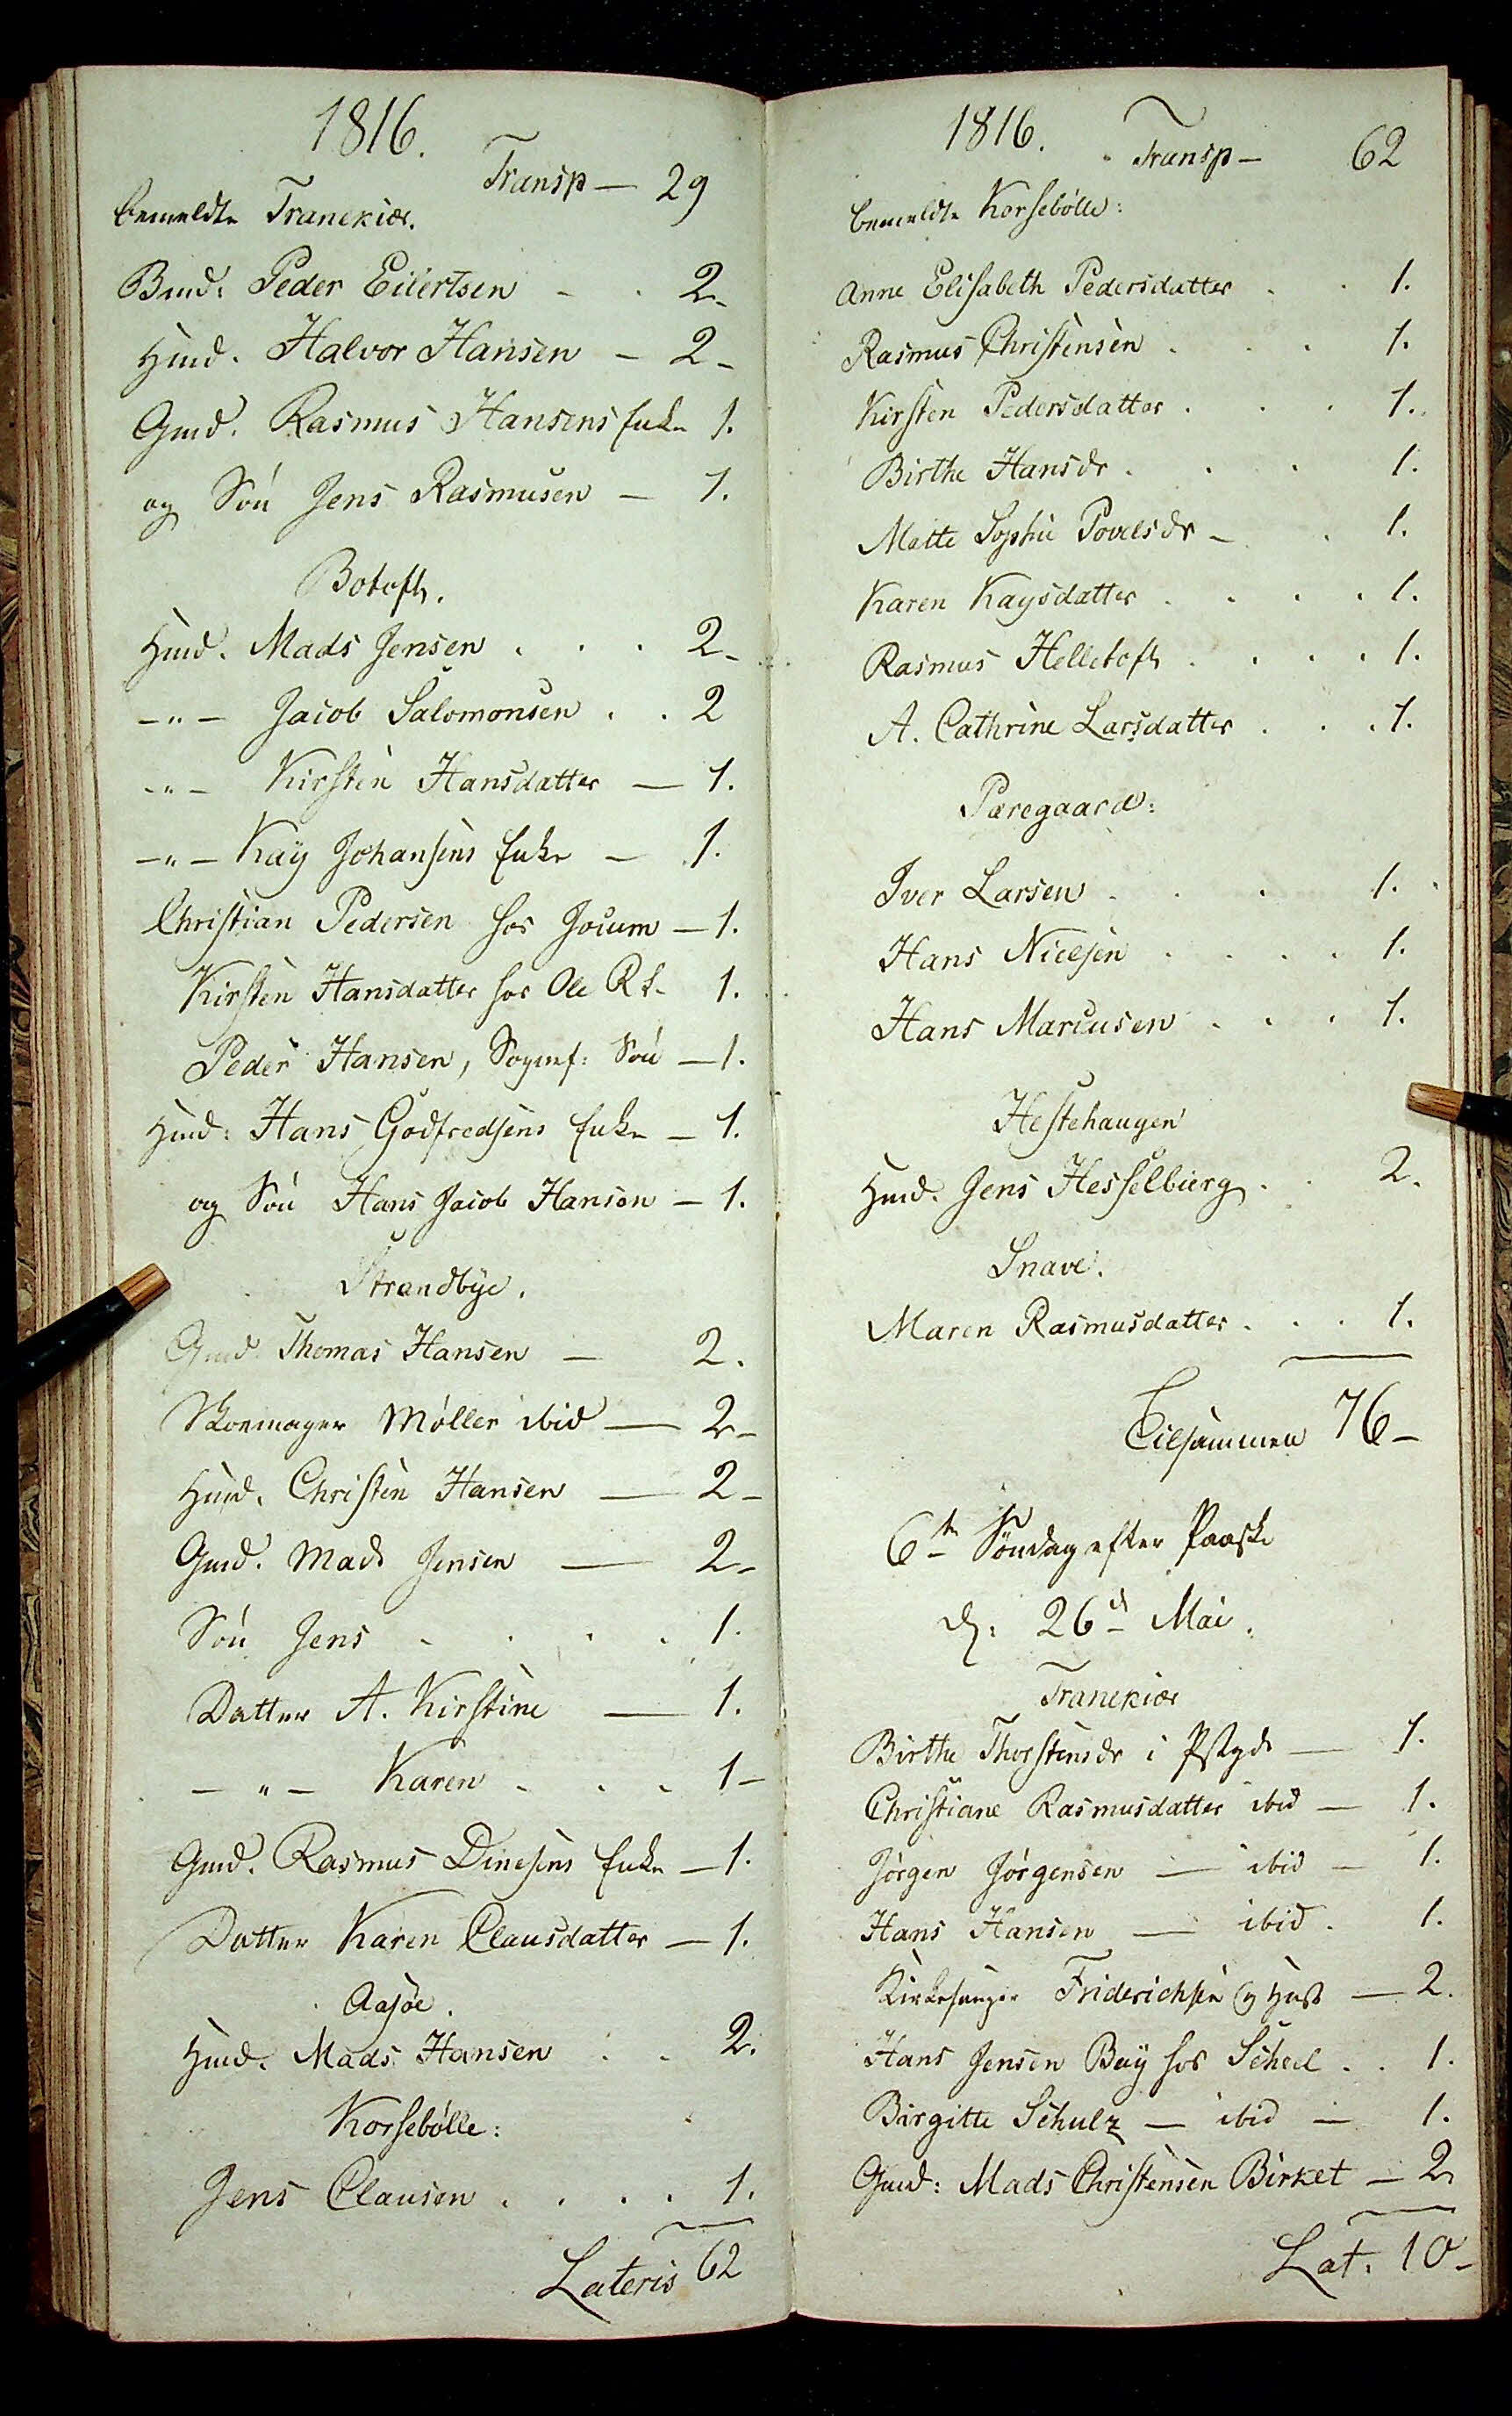1816
Transp - 29
bemeldte Tranekiær.
Bmd: Peder Eilertsen - . 2
Hmd: Halvor Hansen - 2
Gmd. Rasmus Hansens Enke 1.
og Søn Jens Rasmusen - 1.
Botoft.
Hmd. Mads Jensen . . . 2.
-"- Jacob Salomonsen . . 2
-"- Kirsten Hansdatter - 1.
-"- Kay Johansens Enke - 1.
Christian Pedersen hos Jocum -1.
Kirsten Hansdatter hos Ole R## 1.
Peder Hansen, Sognef: Søn - 1.
Hmd: Hans Godfredsens Enke - 1.
og Søn Hans Jacob Hansen - 1.
Strandbye.
Gmd. Thomas Hansen - 2.
Skoemager Møller ibid - 2-
Hmd. Christen Hansen - 2.
Gmd. Mads Jensen - 2
Søn Jens . . . . 1.
Datter A. Kirstine - 1.
-"- Karen . . . 1-
Gmd. Rasmus Dinesens Enke - 1.
Datter Karen Clausdatter - 1.
Aasøe
Hmd. Mads Hansen . . 2.
Korsebølle.
Jens Clausen . . . . 1.
Lateris 62
1816.
Transp - 62
bemeldte Korsebølle:
Anne Elisabeth Pedersdatter . . 1.
Rasmus Christensen . . . 1.
Kirsten Pedersdatter . . . 1.
Birthe Hansdr . . . 1.
Mette Sophie Povelsdr - . 1.
Karen Kaysdatter . . . . 1.
Rasmus Helletoft . . . . 1.
A. Cathrine Larsdatter . . . 1.
Pæregaard:
Iver Larsen . . . 1.
Hans Nielsen . . . . 1.
Hans Marcusen . . . 1.
Hestehaugen
Hmd: Jens Hesselbierg . . 2.
Snave.
Maren Rasmusdatter . . . 1.
Tilsammen 76-
6te Søndag efter Paaske
d: 26de Mai.
Tranekiær
Birthe Thorstensdr i Pstgd - 1.
Christiane Rasmusdatter ibid - 1.
Jørgen Jørgensen - ibid - 1.
Hans Hansen - ibid. 1.
Kirkesanger Friderichsen og Hust - 2.
Hans Jensen Bay hos Scheel . .1.
Birgitte Schulz - ibid - 1.
Gund: Mads Christensen Birket - 2
Lat. 10-
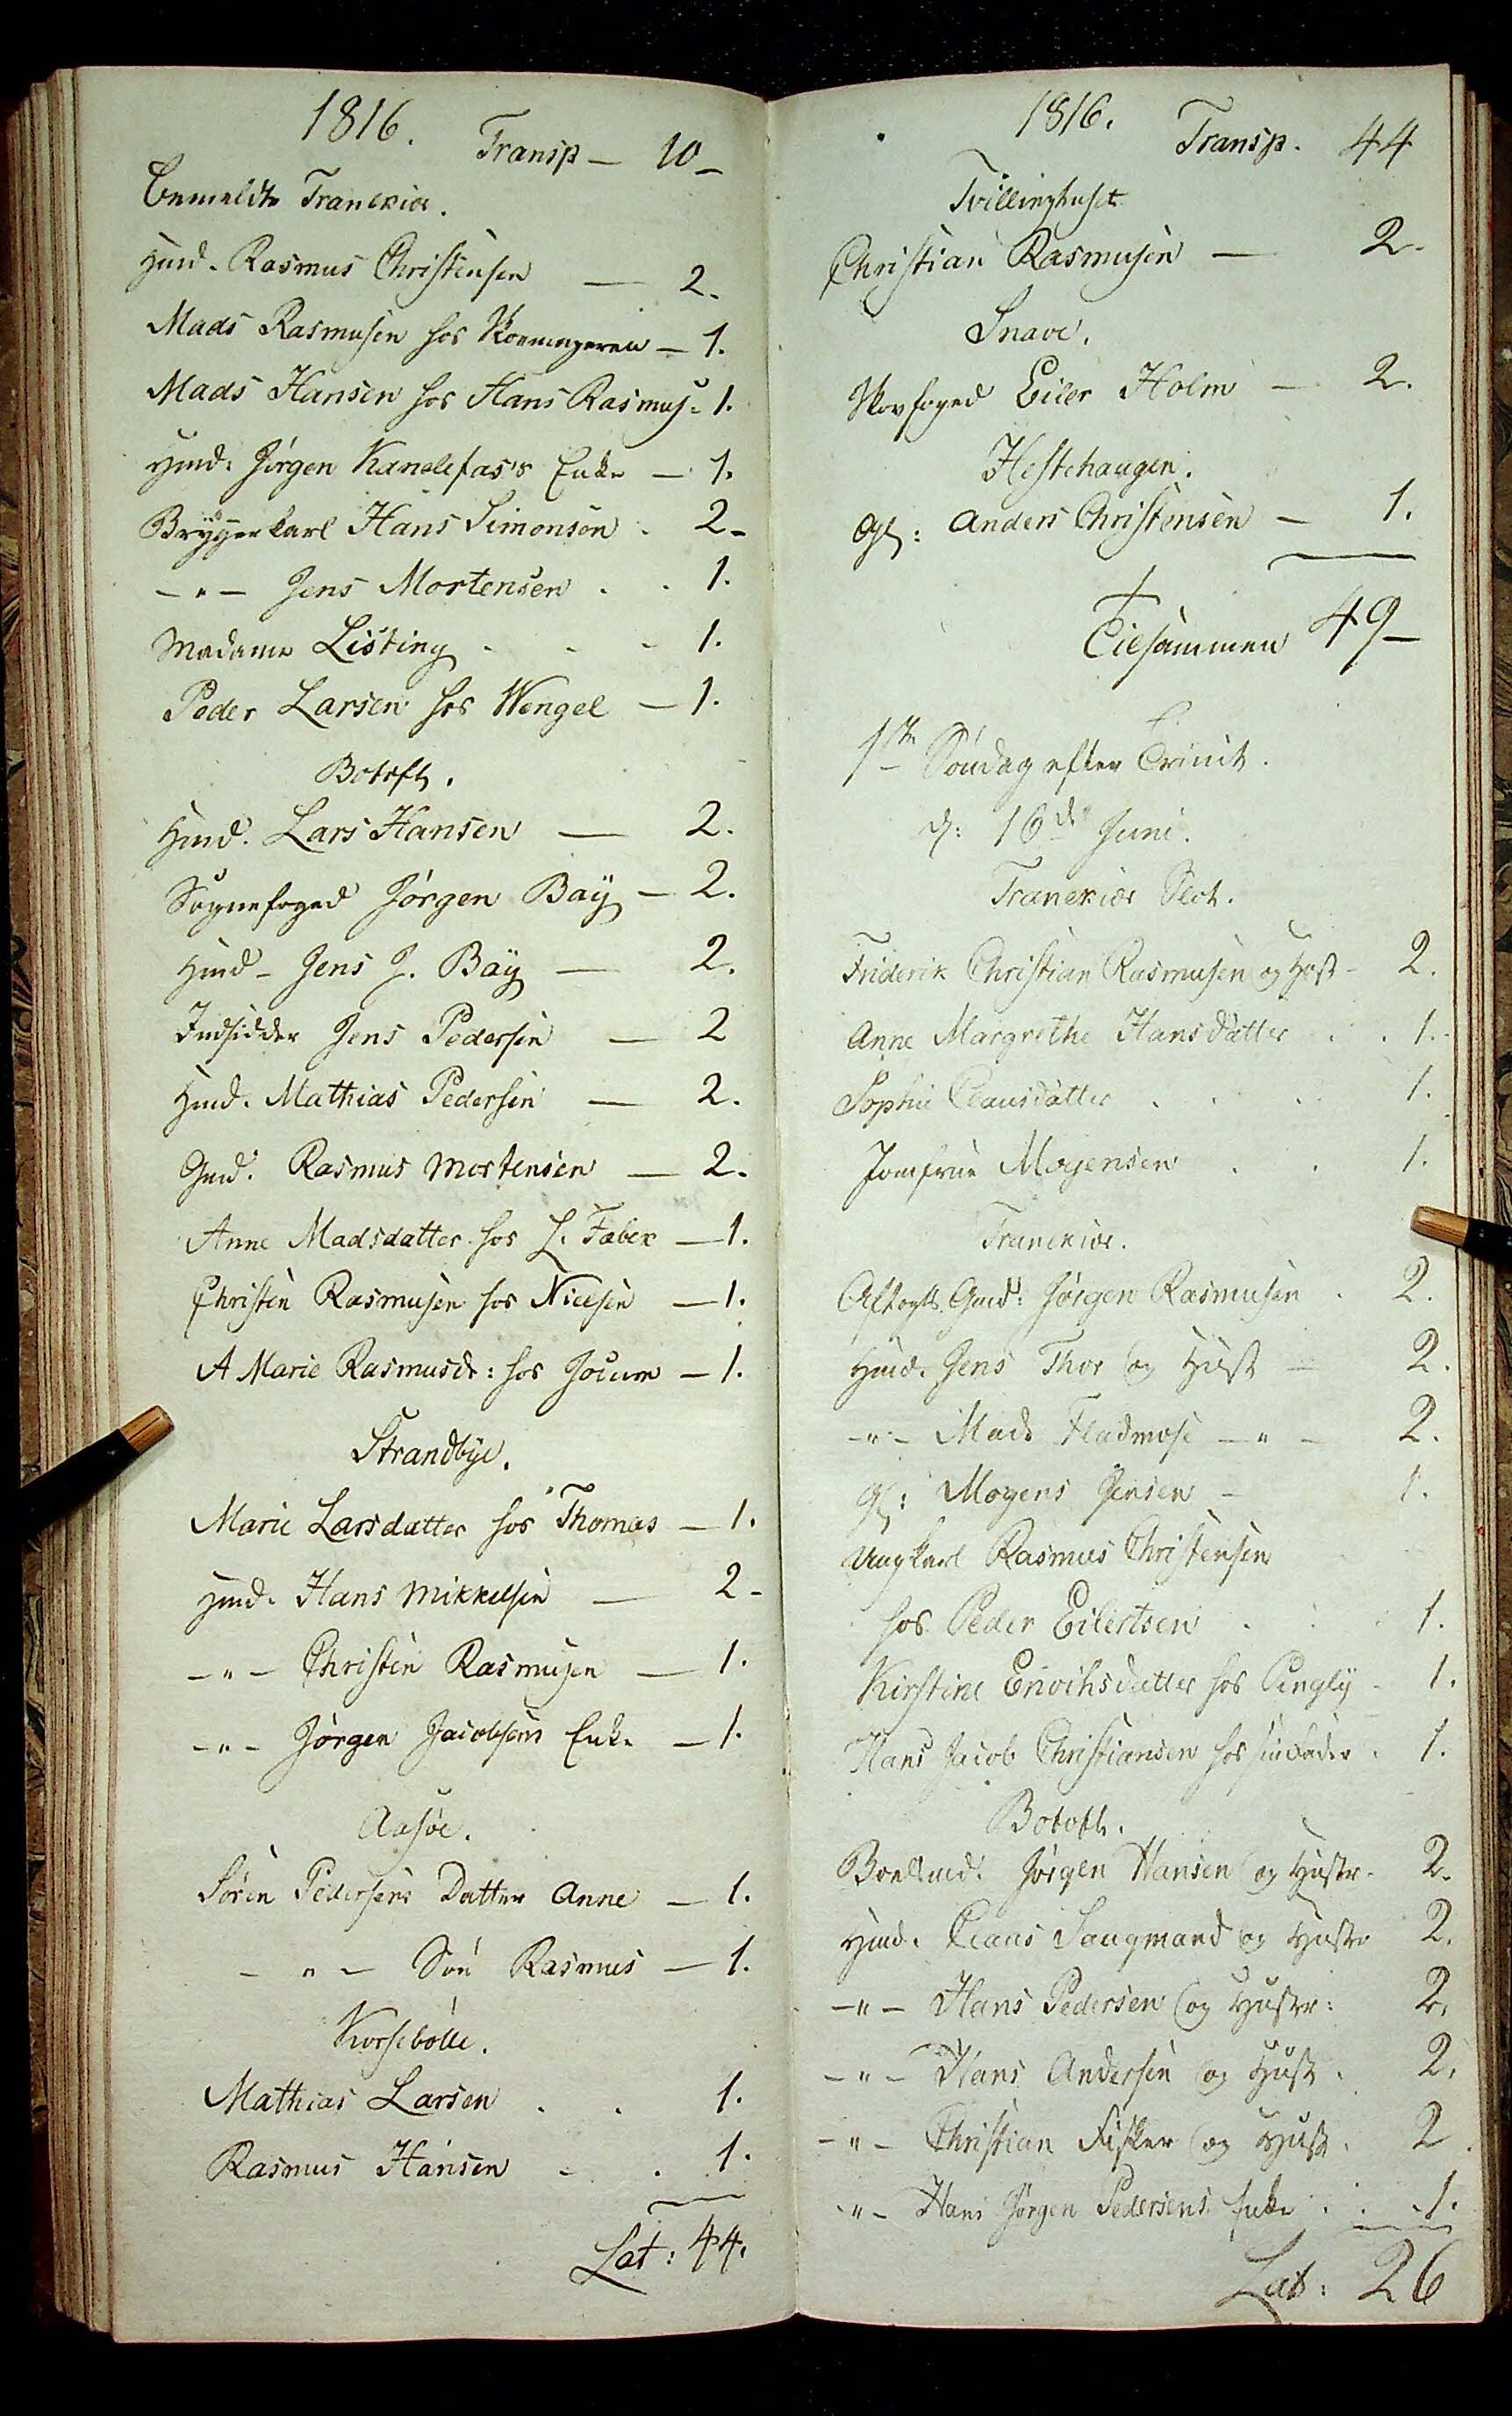1816.
Transp - 10-
bemeldte Tranekiær.
Hmd. Rasmus Christensen - 2
Mads Rasmusen hos Skoemageren - 1.
Mads Hansen hos Hans Rasmus . 1.
Hmd: Jørgen Kanelefas's Enke - 1.
Bryggerkarl Hans Simonsen . 2-
-"- Jens Mortensen . . 1.
Madame Listing . . 1.
Peder Larsen hos Wengel - 1.
Botoft.
Hmd. Lars Hansen - 2.
Sognefoged Jørgen Bay - 2.
Hmd- Jens J. Bay - 2.
Indsidder Jens Pedersen 2
Hmd. Mathias Pedersen - 2.
Grd. Rasmus Mortensen - 2.
Anne Madsdatter hos L. Fæber - 1.
Christen Rasmusen hos Nielsen - 1
A. Marie Rasmusdr: hos Jocum - 1.
Strandbye.
Marie Larsdatter hos Thomas - 1.
Hmd. Hans Mikkelsen - 2
-"- Christen Rasmusen - 1
-"- Jørgen Jacobsens Enke - 1.
Aasøe.
Søren Pedersens Datter Anne - 1.
-"- Søn Rasmus - 1.
Korsebølle.
Mathias Larsen . . 1.
Rasmus Hansen . . 1.
Lat: 44.
1816.
Transp. 44
Tvillinghuset
Christian Rasmusen - 2.
Snave.
Skovfoged Eiler Holm - 2.
Hestehaugen
gl: Anders Christensen - 1.
Tilsammen 49-
1ste Søndag efter Trinit.
d: 18de Juni.
Tranekiær Slot.
Friderik Christian Rasmusen og Hust - 2.
Anne Margrethe HansDatter . . 1.
Sophie Clausdatter . . . . 1.
Jomfrue Mogensen . . 1.
Tranekiær.
Aftægts Gmd: Jørgen Rasmusen . 2.
Hmd. Jens Thor og Hust - 2
-"- Mads Fladmose -"- 2.
Gl: Mogens Jensen - 1.
Ungkarl Rasmus Christensen
hos Peder Eilertsen . . . 1.
Kirstine Enochsdatter hos Pingly . 1.
Man Jacob Christiansens hos sin Fader . 1.
Botoft..
Boelsmd. Jørgen Hansen og Hustr . 2.
Hmd. Claus Saugmand og Hustr 2.
-"- Hans Pedersen og Hustr: 2.
-"- Hans Andersen og Hust . 2.
-"- Christian Fisker og Hust . 2
-"- Hans Jørgen Pedersens Enke . . . 1
Lat: 26